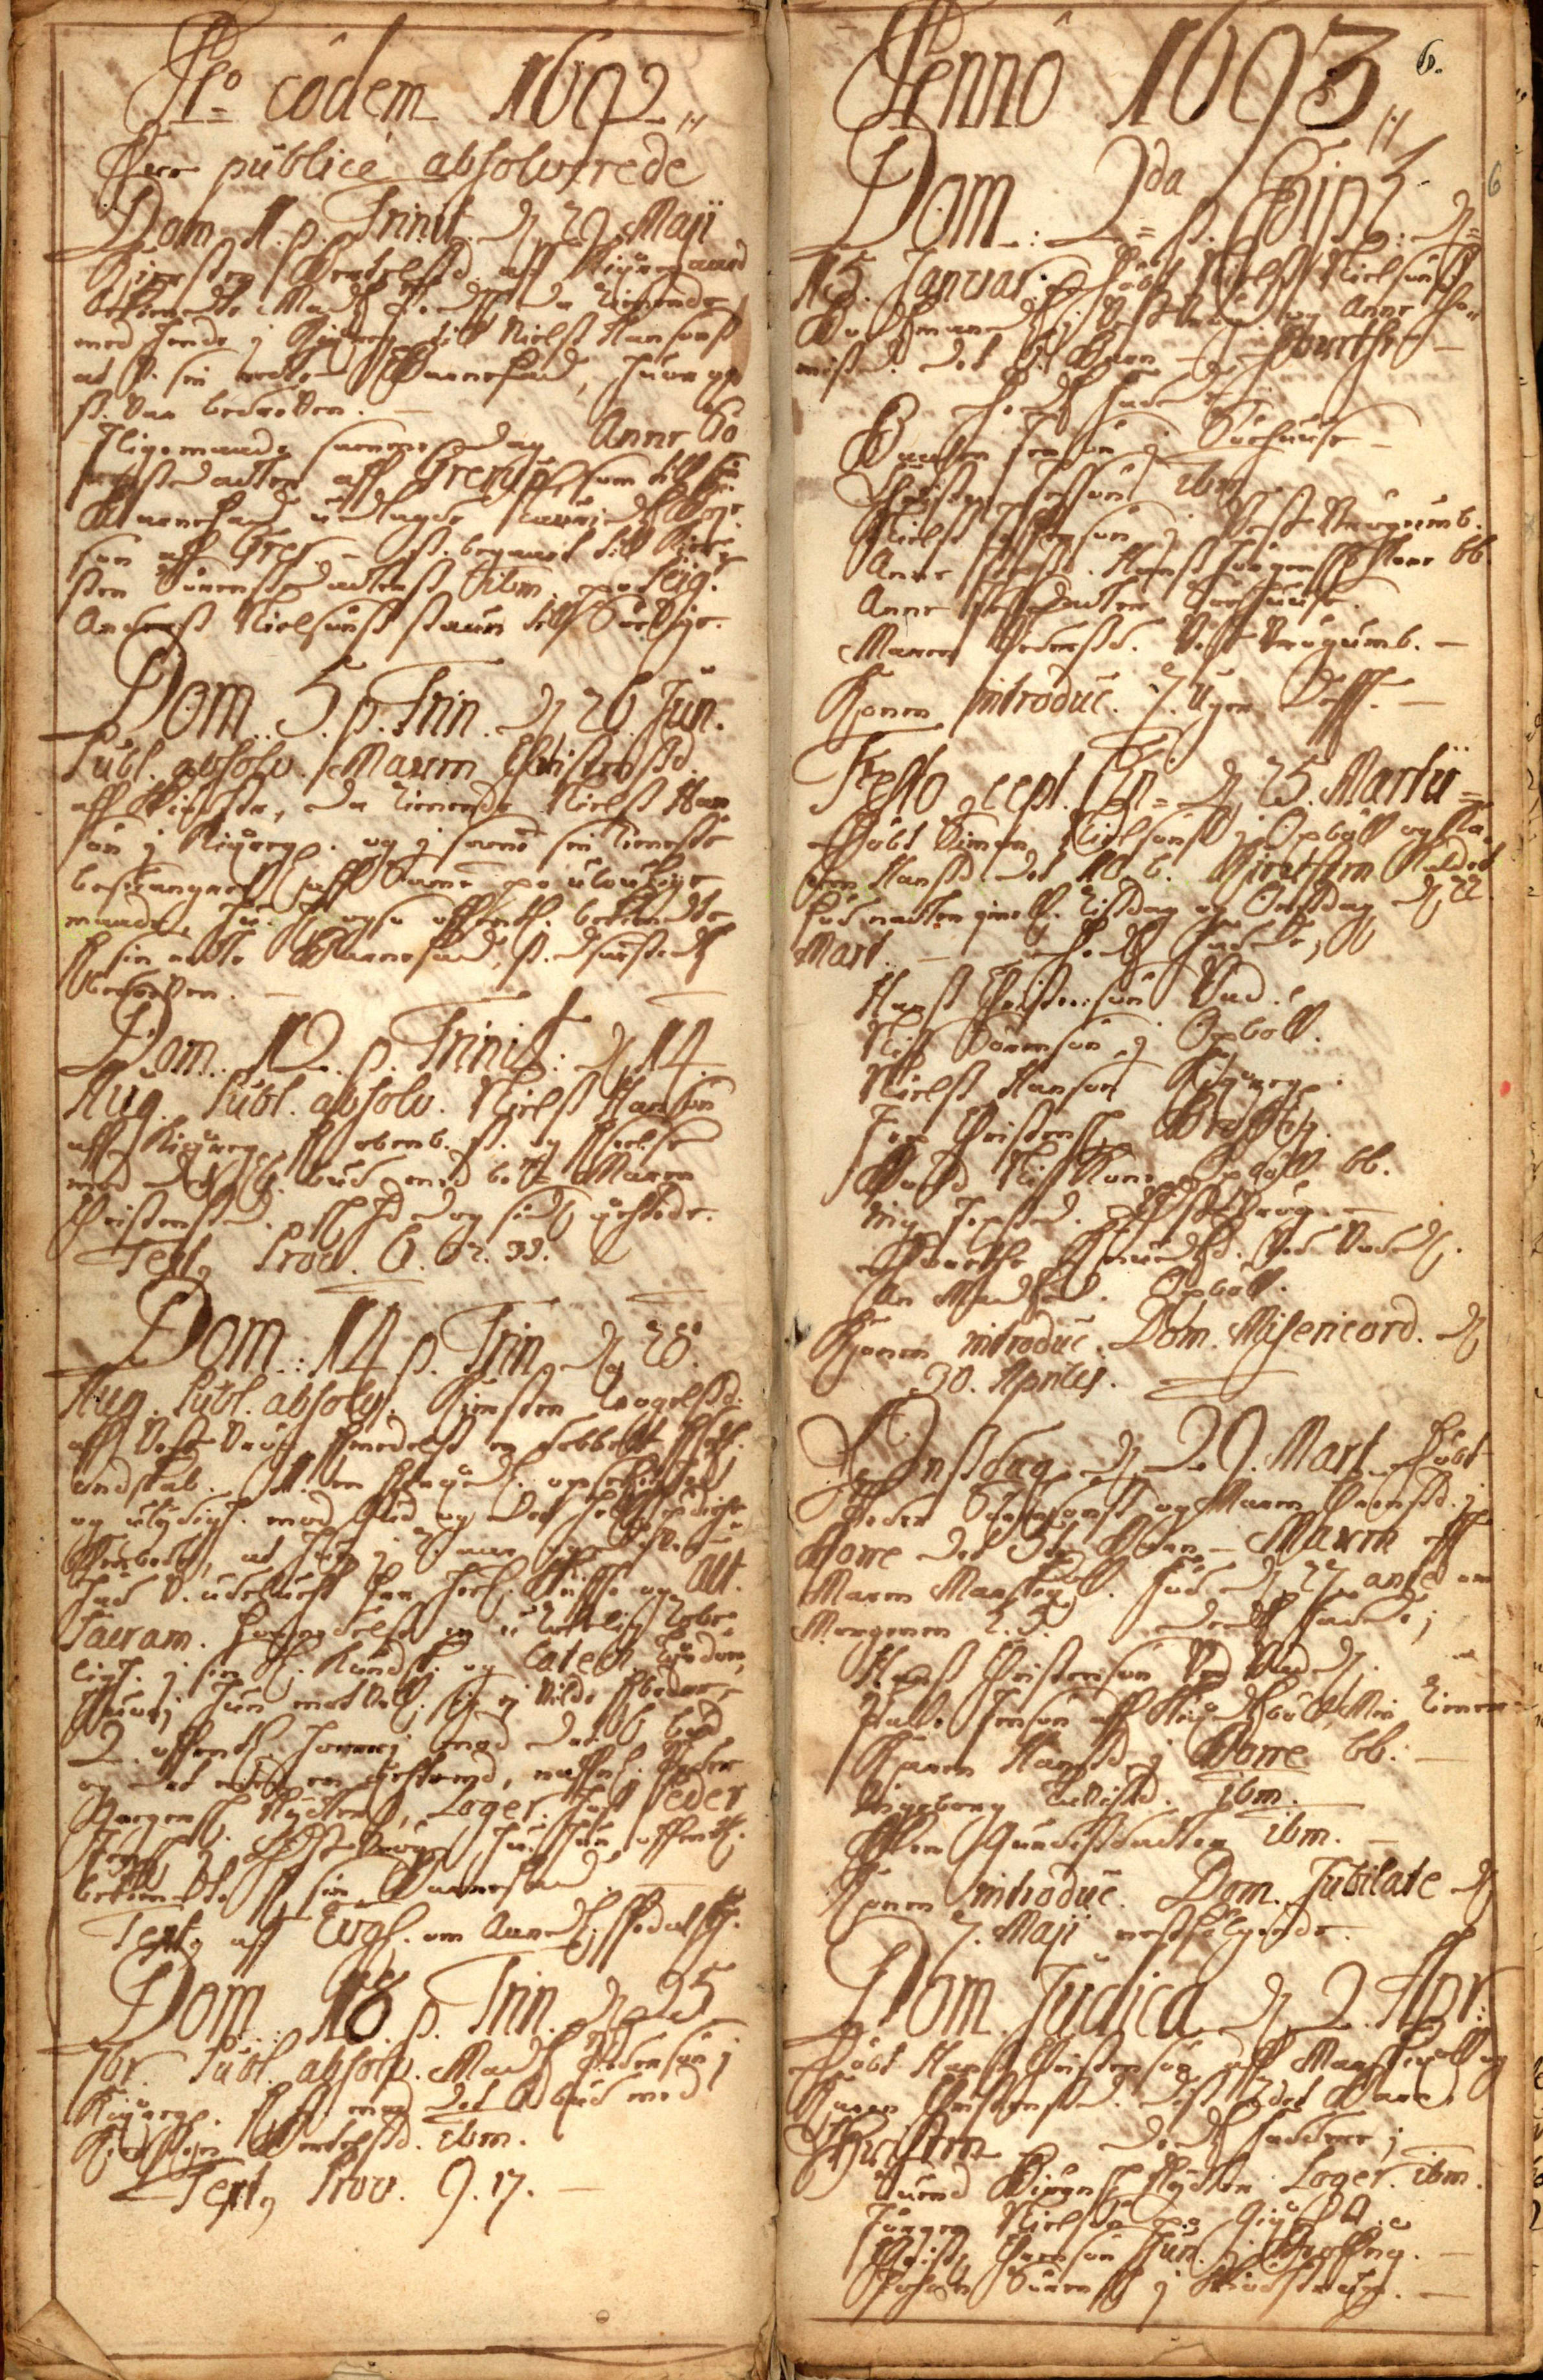Ao eodem 1692,,
Ere publice absolverede
Dom1. p.Trinit. d 29 Maji
Kiersten Bertelsd. aff Kiærgaard
bekiendte Mads Peders da tienende
med hende i Kiærg till Niels Hansøns
at v. sin rette Barnefad, huor og
s. var bedreven.
Iligemaade samme Dag Anne Sø
rensdatter aff Grærup, som till sin
Barnefad udlagde Laurids Boye
sen af Grær- s. begaaet till Kir¬
sten Sørensdatter ibm paa Søg##.
Anders Nielsøns staun till ##.
Dom.. 5. p.Trin. d 26. Jun.
Publ. absolv. Maren Christensd.
aff Skødstr, da tienende Niels Han¬
søn i Kierg##. og j samme sin tieneste
besvangret aff samme paa ulovlige
maade. J## J## ogse offentl. bekjendte
sin rette Barnefad, s. d sammesteds
bedreven.
Dom. 12. p. Trinit: d 14.
Aug. Publ. absolv. Niels Hansen
af Kiæerg## f## obmb## s. og Foelse##
mod det 6. Bud med bem Maren
Christensd. s## Hd dog sid ægtede.
Text. Proc. 6.62.33.
Dom.14 p. Trin. d 28.
Aug. Publ absolv Kiersten Trogelsd.
afd Vester Vrøg, formedelst en dobbelt ##
ondskab. 1. er ## ##
og ulydigh. mod Gud og Det He## ##
## ## ##
## ## ##
Sacram. ## ## ## ut##lig tobe¬
ligh. j sin ## Kundsk. og Catech. Lærdom
## hun ## ## ## sig ej vilde f##
2. offentl. H## mod det 6 Bud
og det med en ##, naffnl. Peder
Jørgenss Rydter, loger. hos Peder
Jenss j Øst Vrøg huor hun offentl.
bekiendte sin Barnefader.
Text aff Evgl. om ## ##
Dom.: 18. p. Trin. d 25.
7br Publ. absolv Mads Pedersen j
Kiærg. f## mod det 6. bud med
Kiersten Bertelsd. ibm.
Text Prov. 9.17.
6
6
Anno 1693
Dom: 2da p. Epiph. d=
15. Januar Døbt Niels Nielsøns
Bodzmand i Vester Vrøg og Anne Tho¬
misd. det 6. Barn Dorethe,
## ## Fadder
Bachen Jensen i Søehuuse
Christen Jessen ibm.
Niels Johansen i Vest Vrøgumb.
Anne Jensd. Hans Jørgenss Kone bb
Anne Jens##datter Søehuuse.
Maren Pedersd. Vest Vrøgumb.
Konen introduc. 7 Uger derefft.
Festo ccpt. Chr. d 25. Martii
Døbt Simon Nielsøns i Oxbøl og Ka¬
ren Hansd det 10 b. Kiersten kaldet
fød natten jmell. Tirsdag og Onsdag d 22
Mart. ## ## Fadde,
Hans Christensøn Vad.
Niss Sørensøn i Oxbøll.
Niels Hansen Kiæreg.
Jep Christens BroEng.
Bueld Niss Kone i Oxbøl bb.
M## Jepsd. Øst Vrøgum.
Dorethe Knudsd. ved Vadd.
An Madsd. Oxbøll.
Konen introduc. Dom. Misericord. d
30. Aprilis.
Onsdag d 29. Mart. Døbt
Peder Sørensøns og Maren Christensd. j
Borre det 5te Barn- Ma## efft
Maren Marskiell fød d 27. ante om
Morgenen k. 5. ## Fadde;
Hans Christensøn ved Vadd.
Palle Jensøn aff H##, Min Tiener
Karen Hansd. j Borre bb.
Ingeborg T##d. ibm.
Ellen Gundisdatter ibm.
Konen introduc. Dom. Jubilate d
7 Maji nestfølgende.
Dom. Judica d 2. Apr:
Døbt Hans Christensøn af Marskiæll og
Karen Christensd. det 2det Barn,
Christen, ## Faddere;
Suend Birgess## Rydter loger. ibm.
Jørgen Nielsen paa Giædb. ##
Christen Christensen Jun. i BroEng.
Johan Sørenss j Skødstrup.